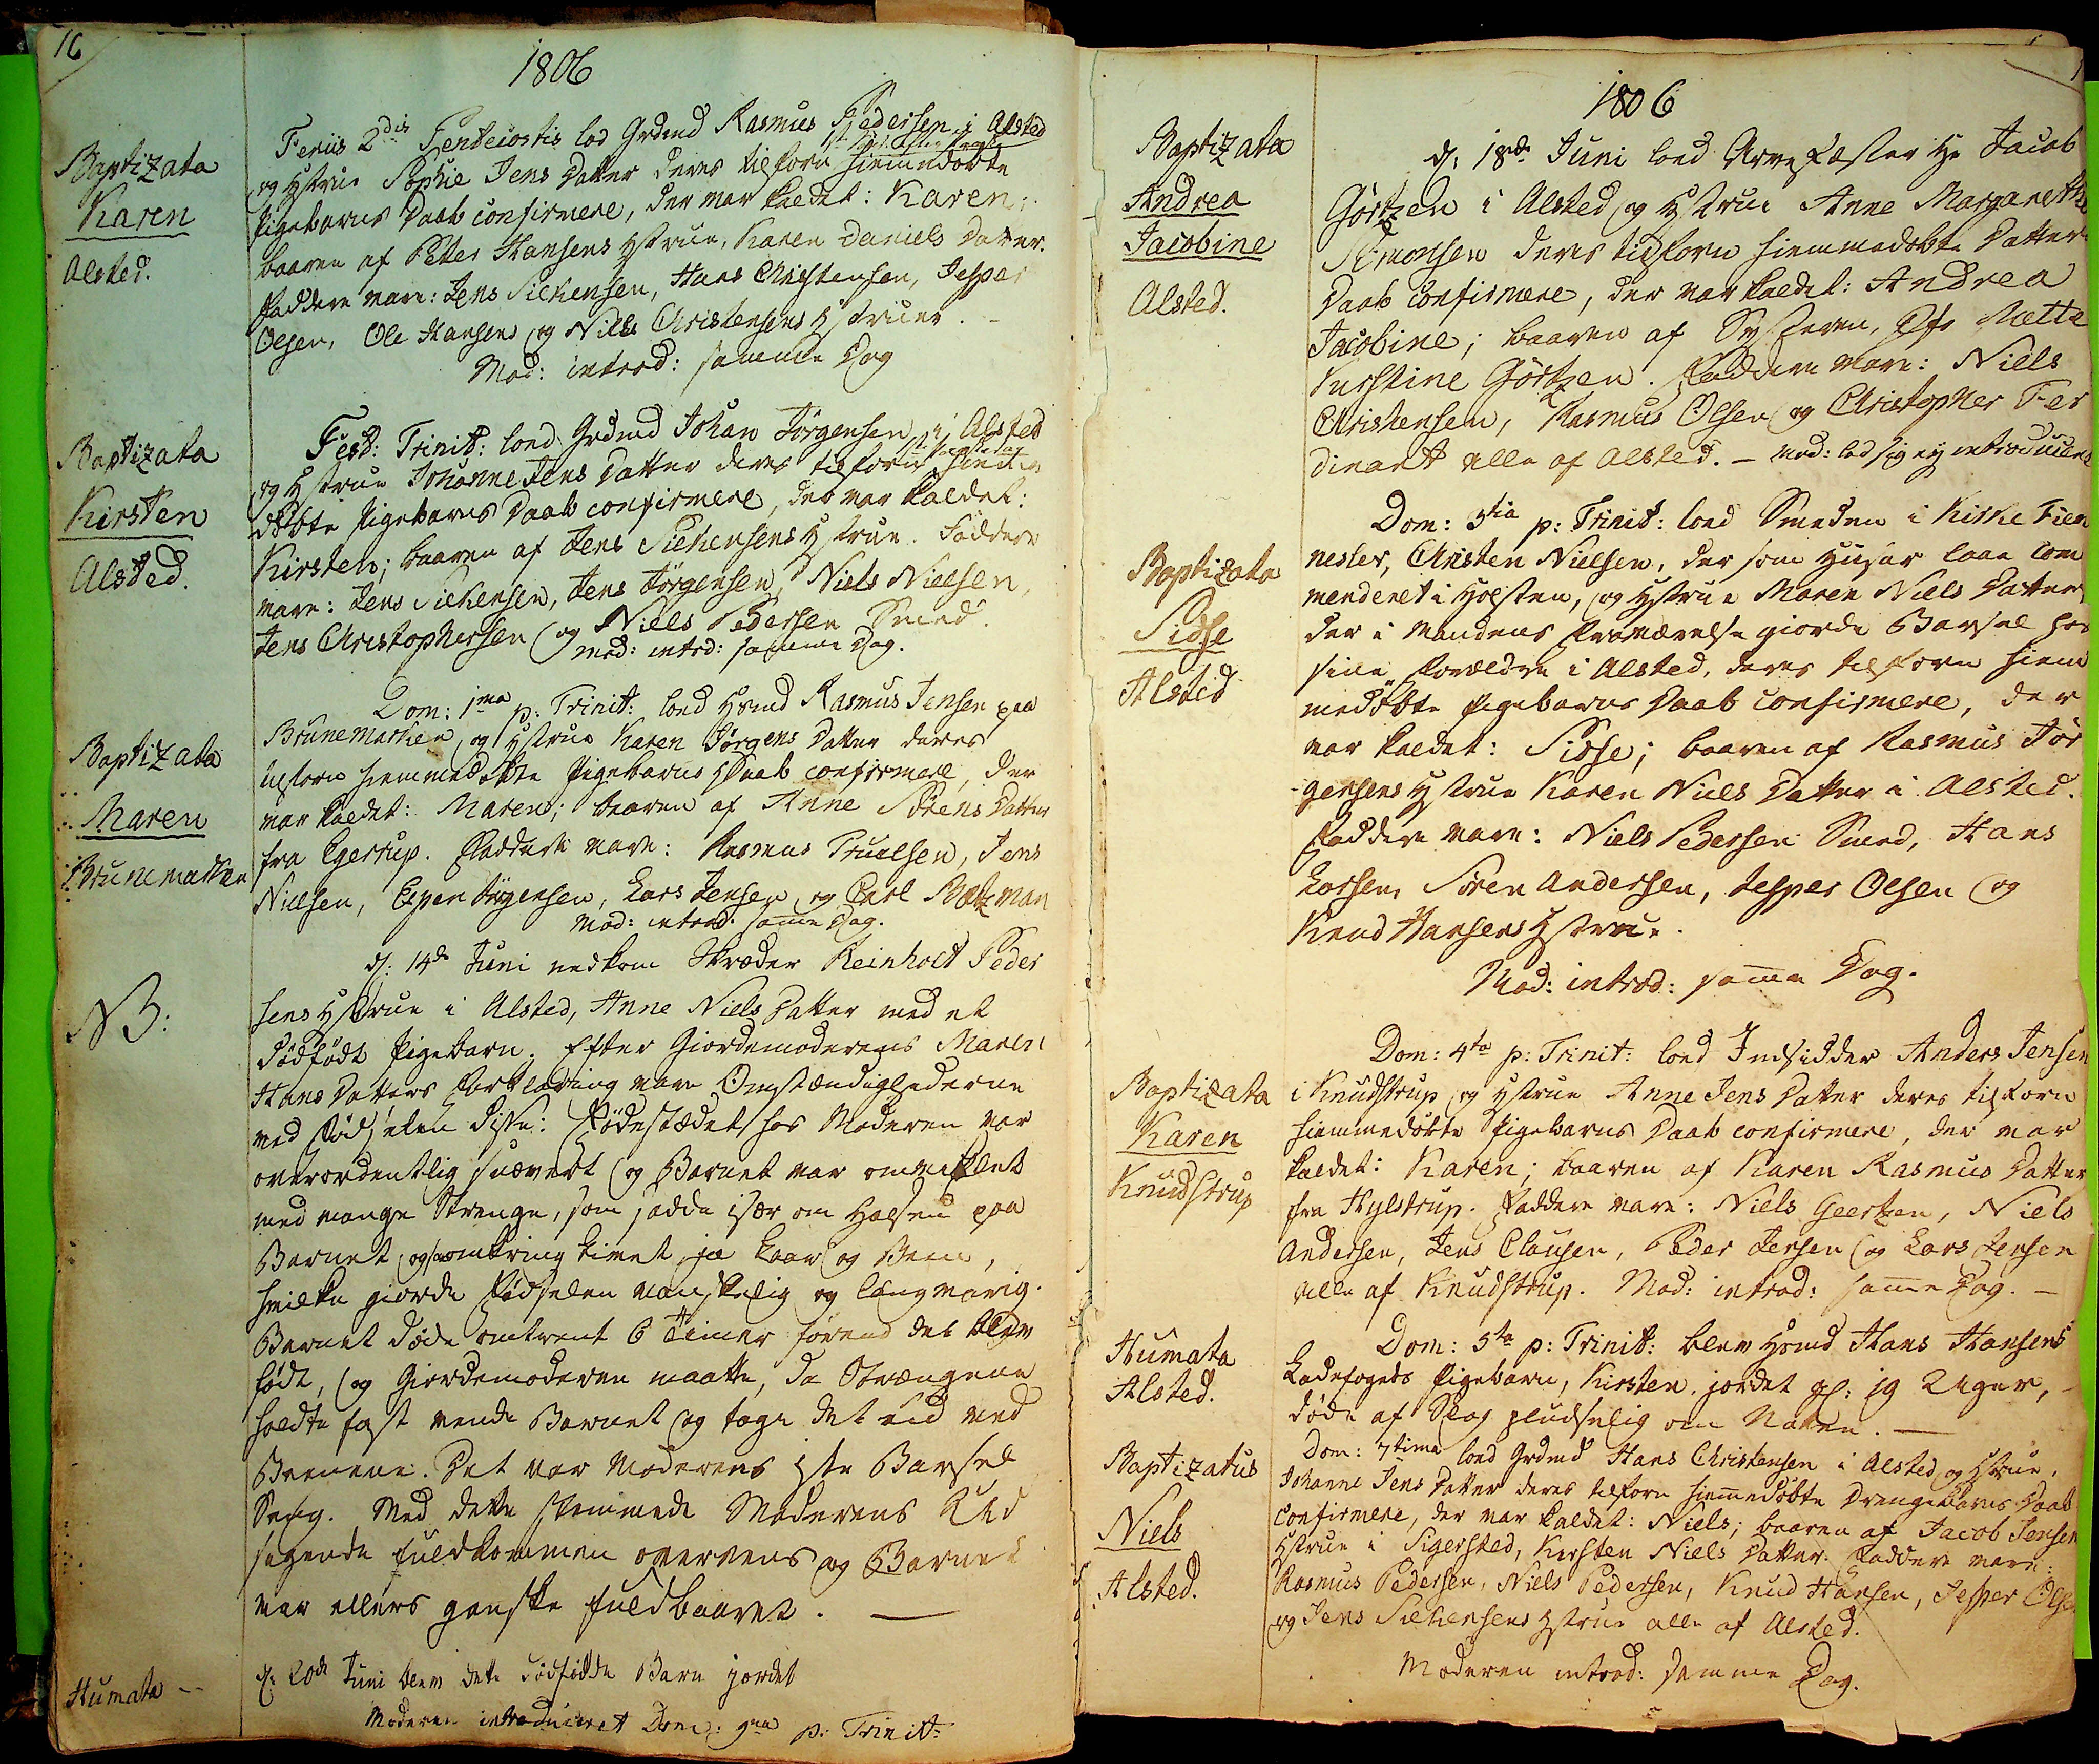16
Baptizata
Karen
Alsted.
Baptizata
Kirsten
Alsted
Baptizata
Maren
Brunemarken
NB:
Humata
1806.
Feriis 2den Pentecostis lod Grdmd Rasmus Pedersen i Alsted
1ste Sønd: efter Paaske
og Hstrue Sophie Jens Datter deres tilforn hiemmedøbt
Pigebarns Daab confirmere, der var kaldet: Karen;
baaren af Peter Hansens Hstrue, Karen Daniels Datter.
Faddere vare: Jens Silkensen, Hans Christensen, Jesper
Olsen, Ole Hansens og Niels Christensens Hstrue
Mod: introd: samme Dag
Fest: Trinit: loed Grdmd Johan Jørgensen i Alsted
1ste Paaskedag
og Hstrue Johanne Jens Datter deres tilforn hiemme¬
døbte Pigebarns Daab confirmere, der var kaldet:
Kirsten; baaren af Jens Silkensens Hstrue. Faddere
vare: Jens Silkensen, Jens Jørgensen Niels Nielsen
Jens Christophersen og Niels Pedersen Smed.
Mod: introd: samme Dag.
Dom: 1ma p: Trinit: loed Hsmd Rasmus Jensen paa
Brunemarken og Hstrue Karen Jørgens Datter deres
tilforn hiemmedøbte Pigebarns Daab confirmere, der
var kaldet: Maren; baaren af Anne Sørens Datter
fra Egerrup. Faddere vare: Rasmus Truelsen, Jens
Nielsen, Espen Jørgensen, Lars Jensen og Carl Bætzman
Mod: introd: samme Dag.
d: 14de Juni nedkom Skræder Reinholt Peder
sens Hstrue i Alsted, Anne Niels Datter med et
dødfødte Pigebarn. Efter Giordemoderens Maren
Hans Datters Forklaring vare Omstændighederne
ved fødselen disse: Fødestædet hos Moderen var
overordentlig snævert og Barnet var omviklet
med mange Strenge, som sadde især om Halsen paa
Barnet, og omkring livet ## laar og Been,
hvilke giorde Fødselen vanskelig og langvarig.
Barnet døde omtrent 6 Timer førend det blev
født, og Giordemoderen maatte, da Strængene
holdte fast vende Barnet og tage det ud ved
Beenene. Det var Moderens 1ste Barsel
Seng. Med dette stemmed Moderens Ud
segende fuldkommen overens og Barnet
var ellers ganske fuldbaaret.
d. 20de Juni blev det dødfødte Barn jordet
Moderen introduceret Dom: 9na p: Trinit:
Baptizata
Andrea
Jacobine
Alsted.
Baptizata
Sidse
Alsted.
Baptizata
Karen
Knudstrup
Humata
Alsted
Baptizatus
Niels
Alsted.
1806
d: 18de Juni loed Arvefæster Hr Jacob
Gørtzen i Alsted og Hustrue Anne Margarethe
Simonsen deris tilforn hiemmedøbte Datters
Daab confirmere, der var kaldet: Andrea
Jacobine; baaren af Systeren, Jfr Mætte
Kirstine Gørtzen. Faddere vare: Niels
Christensen, Rasmus Olsen og Christopher Fer
dinant alle af Alsted. - Mod: lod sig ey introducere
Dom: 3tia p: Trinit: loed Smeden i Kirke Fien
neslev, Christen Nielssen, der som Husar laae com
menderet i Holsten, og Hstrue Maren Niels Datter
der Mandens Fraværelse giorde Barsel hos
sine Forældre i Alsted, deres tilforn hiem
medøbte Pigebarns Daab confirmere, der
var kaldet: Sidse; baaren af Rasmus Jør
gensens Hstrue Karen Niels Datter i Alsted.
Faddere vare: Niels Pedersen Smed, Hans
Larsen Søren Andersen, Jesper Olsen og
Knud Hansens Hstrue.
Mod: introd: samme Dag.
Dom: 4ta p: Trinit: lod Indsidder Anders Jensen
i Knudstrup og Hstrue Anne Jens Datter deres tilforn
hiemmedøbte Pigebarns Daab confirmere, der var
kaldet: Karen; baaren af Karen Rasmus Datter
fra Hylstrup. Faddere vare: Niels Geertzen, Niels
Andersen, Jens Clausen, Peder Jensen og Lars Jensen
alle af Knudstrup. Mod: introd: samme Dag.
Dom: 5te p: Trinit: blev Hsmd Hans Hansens
Ladefogeds Pigebarn, Kirsten. jordet gl: 19 Uger,
døde af Slag pludselig om Natten.
Dom: 7tima loed Grdmd Hans Christensen i Alsted, og Hstrue
Johanne Jens Datter deres tilforn hiemmedøbte Drengebarns Daab
confirmere, der var kaldet: Niels; baaren af Jacob Jensen
Hstrue i Sigersted, Kirsten Niels Datter: Faddere vare:
Rasmus Pedersen, Niels Pedersen, Knud Hansen, Jesper Olsen
og Jens Silkensens Hstrue alle af Alsted
Moderen introd: samme Dag.
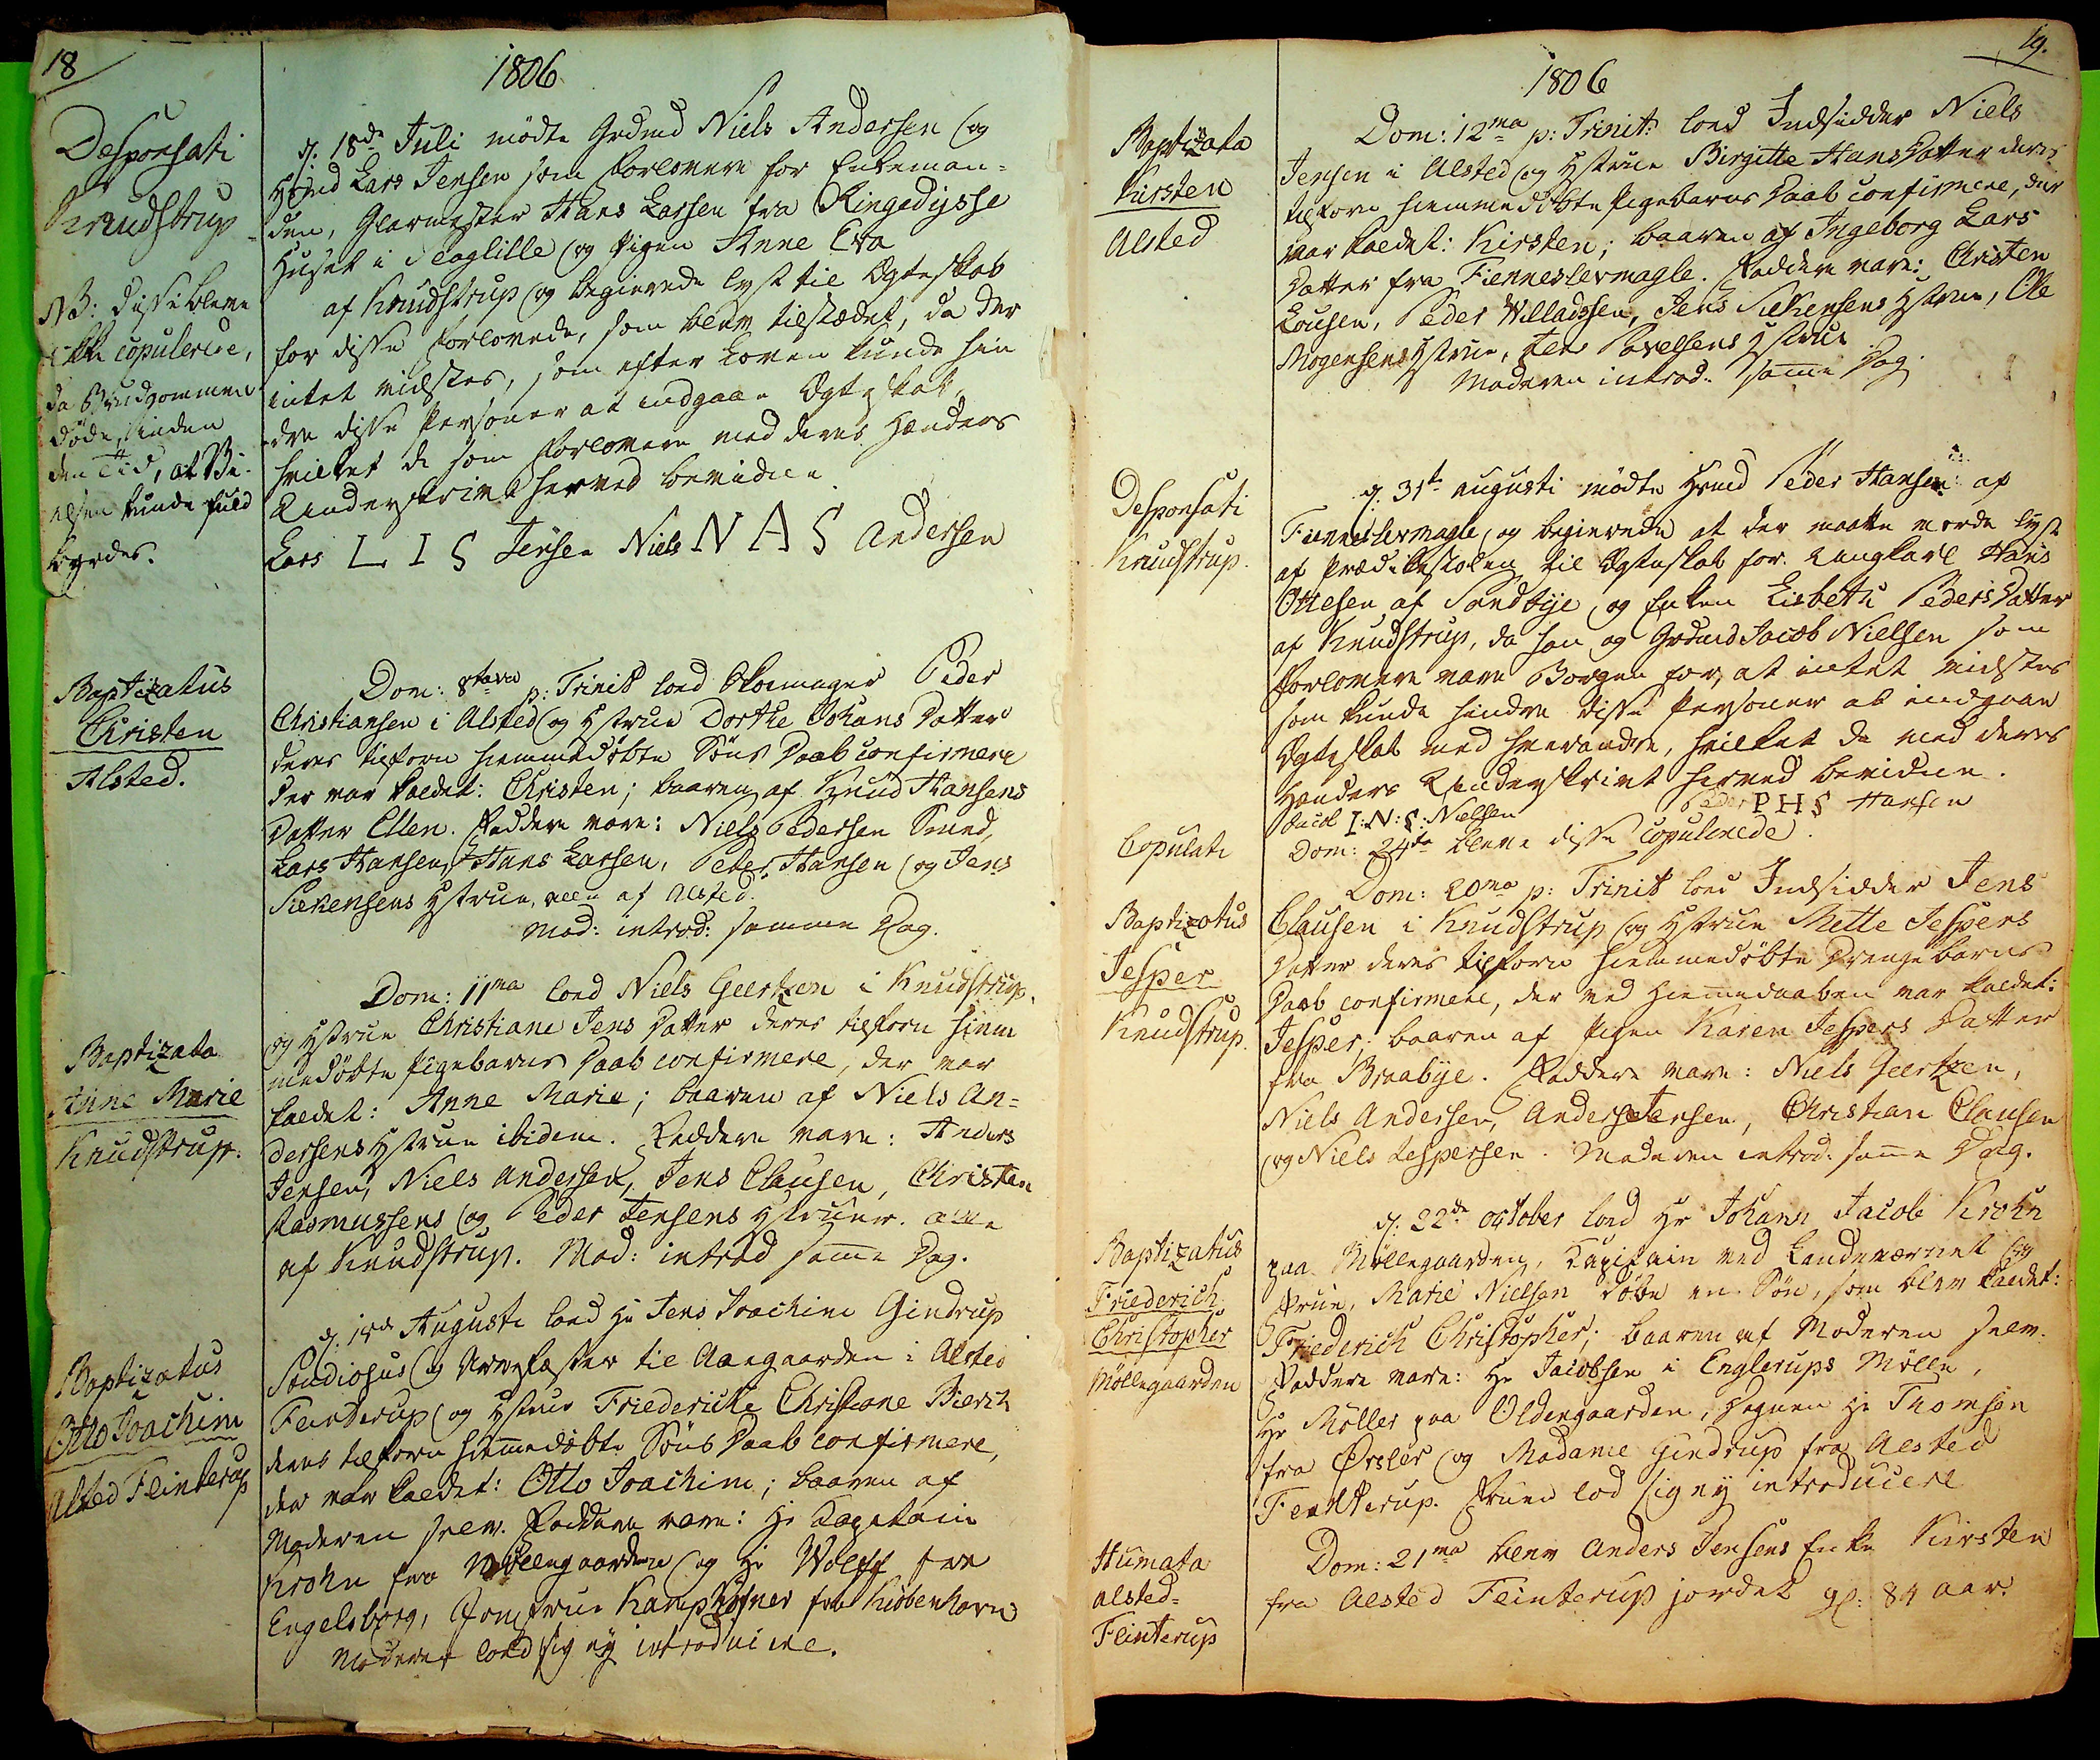18
Desponsati
Knudstrup
NB: disse bleve
ikke copulerede,
da Brudgommen
døde inden
den Tid, at Vi¬
elsen kunde fuld
byrdes.
Baptizatus
Christen
Alsted.
Baptizata
Anne Marie
Knudstrup.
Baptizatus
Otto Joachim
Alsted Flinterup
1806
d: 18de Juli mødte Grdmd Niels Andersen og
Hsmd Lars Jensen som forlovere for Enkeman¬
den, Glarmester Hans Larsen fra Ringedysse
Huset i Slaglille og Pigen Anne Eva
af Knudstrup og begierede lyst til Ægteskab
for disse Forlovede, som blev tilstædet, da der
intet vidstes, som efter Loven kunde hin
dre disse Personer at indgaae Ægteskab,
hvilket de som Forlovere med deres Hænders
Underskrivt herved bevidne
Lars L I S Jensen Niels N A S Andersen
Dom: 8tava p: Trinit loed Skoemager Peder
Christiansen i Asted og Hstrue Dorthe Johans Datter
deres tilforn hiemmedøbte Søns Daab confirmere
der var kaldet: Christen; baaren af Knud Hansens
Datter Ellen. Faddere vare: Niels Pedersen Smed
Lars Hansens, Hans Larsen, Peder Hansen og Jens
Silkensens Hstrue, alle af Asted.
Mod: introd: samme Dag.
Dom: 11ma loed Niels Geertzen i Knudstrup
og Hstrue Christiane Jens Datter deres tilforn hiem
medøbte Pigebarns Daab confirmere, der var
kaldet: Anne Marie; baaren af Niels An¬
dersens Hstrue ibidem. Faddere vare; Anders
Jensen, Niels Andersen, Jens Clausen, Christen
Rasmussens og Peder Jensens Hstruer. Alle
af Knudstrup. Mod: introd samme Dag.
d: 14de Augusti loed Hr Jens Joachim Gindrup
Studiosus og Arvefæster til Aaegaarden i Alsted
Flinterup og Hstue Frideriche Christiane Bierch
deres tilforn hiemmedøbte Søns Daab confirmere,
der var kaldet: Otto Joachim; baaren af
Moderen selv. Faddere vare: Hr Kapetain
Krohn fra Møllegaarden og Hr Wolff fra
Engelsborg, Jomfrue Kamphøfner fra Kiøbenhavn
Moderen loed sig ey introducere.
Baptizata
Kirsten
Alsted
Desponsati
Knudstrup.
Copulati
Baptizatus
Jesper
Knudstrup
Baptizatus
Friederich
Christopher
Møllegaarden
Humata
Alsted
Flinterup
19.
1806
Dom: 12ma p: Trinit: loed Indsidder Niels
Jensen i Alsted og Hstrue Birgitte Hans Datter deres
tilforn hiemmedøbte Pigebarns Daab confirmere, der
var kaldet: Kirsten; baaren af Ingeborg Lars
Datter fra Fiennestevmagle. Faddere vare: Christen
Lausen, Peder Willadsen, Jens Silkensens Hstrue, Ole
Mogensens Hstrue, Jens Povelsens Hstrue
Moderen introd: samme Dag
d: 31te Augusti mødte Hsmd Peder Hansen af
Fienneslevmagle og begierede at der maatte vorde lyst
af Prædikestolen til Ægteskab for Ungkarl Hans
Ottesen af Sandbye og Enken Lisbeth Peders Datter
af Knudstrup, da han og Grdmd Jacob Nielsen som
Forlovere vare Borgen for at intet vidstes
som kunde hindre disse Personer at indgaae
Ægteskab med hverandre, hvilket de ved deres
Hænders Underskrivt herved bevidne
Jacob I: N: S: Nielsen
Peder P H S Hansen
Dom: 24de blev disse copulerede
Dom: 20ma p: Trinit loed Indsidder Jens
Clausen i Knudstrup og Hstrue Mette Jespers
Datter deres tilforn hiemmedøbte Drengebarns
Daab confirmere, der ved Hiemmedaaben var kaldet:
Jesper, baaren af Pigen Karen Jespers Datter
fra Braabye. Faddere vare: Niels Geertzen,
Niels Anderssen, Anders Jensen, Christian Clausen
og Niels Jespersen. Moderen introd: samme Dag.
d. 22de october loed Hr Johann Jacob Krohn
paa Møllegaarden, Kapitain ved Landeværnet og
Frue, Marie Nielsen døbe en Søn, som blev kaldet:
Friederich Christopher, baaren af Moderen selv.
Faddere vare: H Jacobsen i Englerup Mølle,
Hr Møller paa Oldengaarden, Degnen Hr Thomsen
fra Ørslev og Madame Gindrup fra Alsted
Flinterup. Fruen lod sig ey introducere
Dom: 21ma blev Anders Jensens Enke Kirsten
fra Alsted Flinterup jordet gl: 84 Aar.